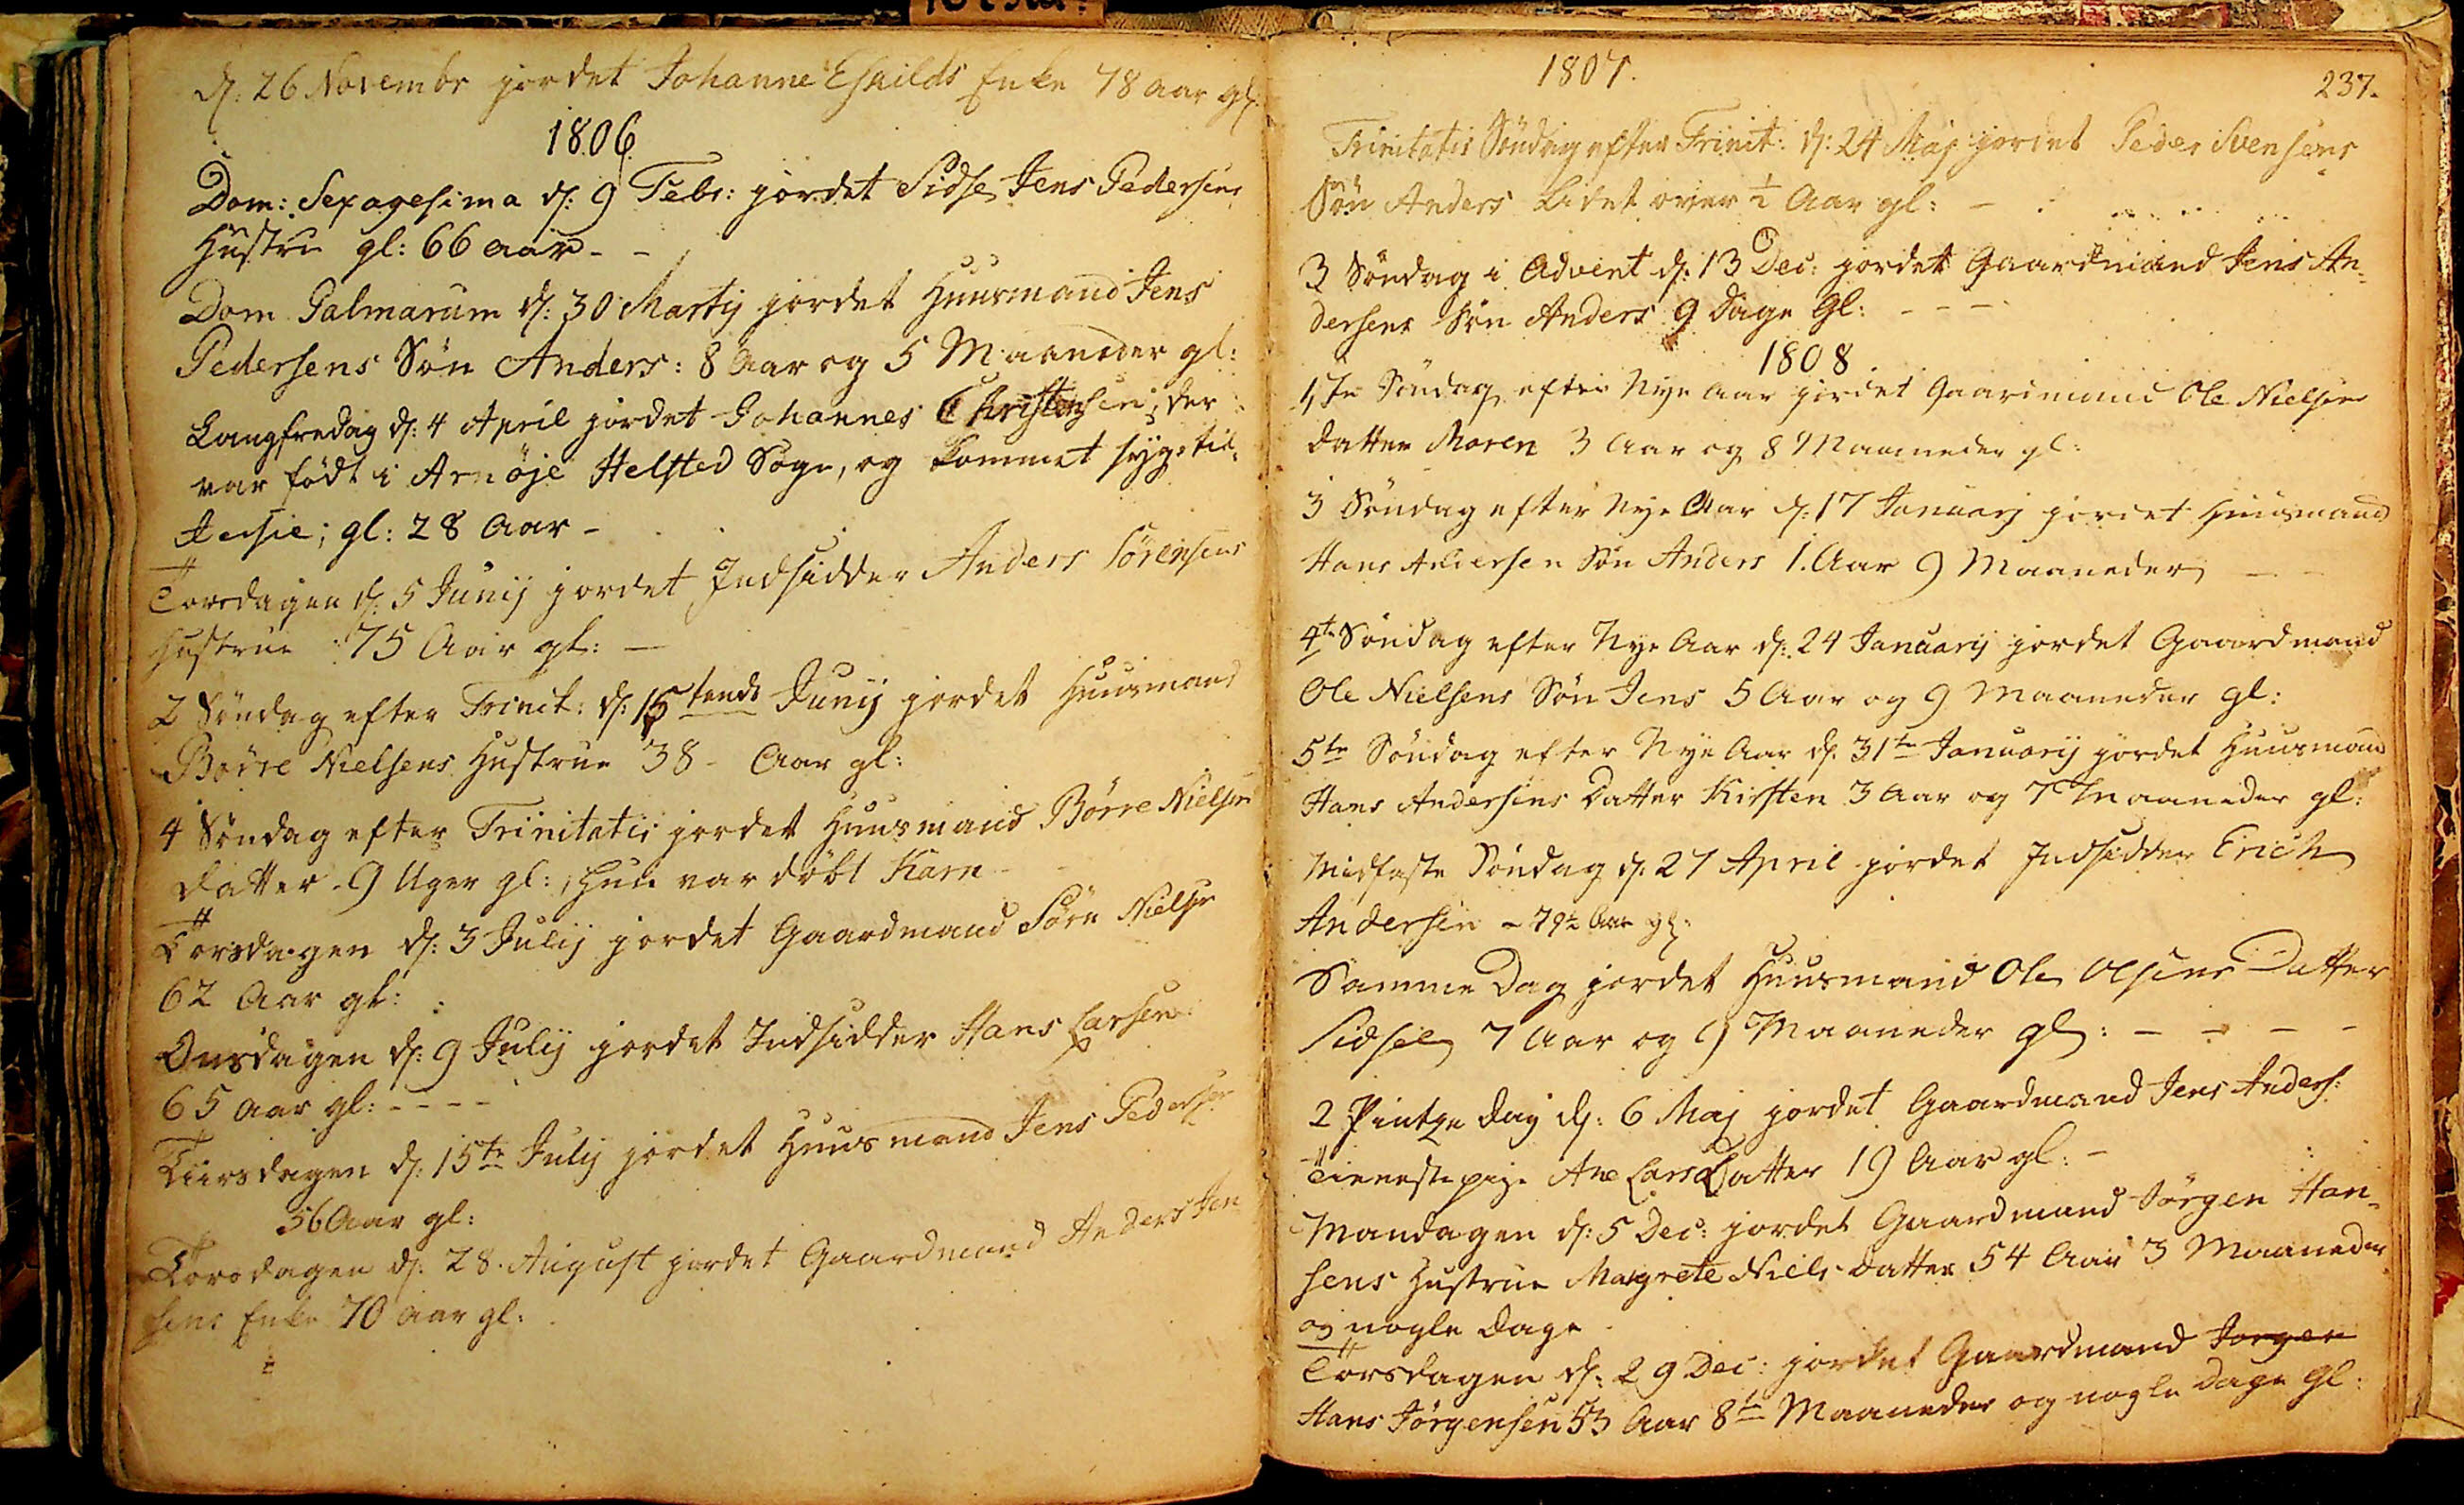d: 26 Novembr jordet Johanne Eskilds Enke 78 Aar gl:
1806
Dom: Sexagesima d: 9 Febr: jordet Sidse Jens Pedersens
Hustru gl: 66 Aar
Dom Palmarum d: 30 Martij jordet Huusmand Jens
Pedersens Søn Anders: 8 Aar og 5 Maaneder gl:
Langfredag d: 4 April jordet Johannes Christensen; der
var født i Arnøje Helsted Sogn, og kommet syg til
Jersie; gl: 28 Aar
Torsdagen d: 5 Junij jordet Indsidder Anders Sørensens
Hustrue 75 Aar gl:
2 Søndag efter Trinit: d: 15tende Junij jordet Huusmand
Børre Nielsens Hustrue 38 Aar gl:
4 Søndag efter Trinitatis jordet Huusmand Børre Nielsens
Datter 9 Uger gl:; hun var døbt Karn
Torsdagen d: 3 Julij jordet Gaardmand Sørn Nielsen
62 Aar gl:
Onsdagen d: 9 Julij jordet Indsidder Hans Larsen
65 Aar gl: 
Tiirsdagen d: 15te Julij jordet Huusmand Jens Pedersen
56 Aar gl:
Torsdagen d: 28 August jordet Gaardmand Anders Jen
sens Enke 70 Aar gl:
237.
1807
Trinitatis Søndag efter Trinit: d: 24 Maj jordet Peder Svensens
Søn Anders Lidet over ½ Aar gl: -
3 Søndag i Advent d: 13 Dec: jordet Gaardmand Jens An¬
dersens Søn Anders 9 Dage gl:
1808.
1ste Søndag efter Nye Aar jordet Gaardmand Ole Nielsens
Datter Maren 3 Aar og 8 Maaneder gl:
3 Søndag efter Nye Aar d: 17 Januarj jordet Huusmand
Hans Andersen Søn Anders 1. Aar 9 Maneder
4te Søndag efter Nye Aar d: 24 Januarij jordet Gaardmand
Ole Nielsens Søn Jens 5 Aar og 9 Maaneder gl:
5te Søndag efter Nye Aar d. 31 Januarij jordet Huusmand
Hans Andersens Datter Kirsten 3 Aar og 7 Maaneder gl:
Midfaste Søndag d: 27 April jordet Indsidder Erich
Andersen - 79½ Aar gl:
Samme Dag jordet Huusmand Ole Olsens Datter
Sidsel 7 Aar og 9 Maaneder gl:
2 Pintze Dag d: 6 Maj jordet Gaardmand Jens Anders:
Tienestepige Ane Lars Datter 19 Aar gl:
Mandagen d: 5 Dec: jordet Gaardmand Jørgen Han¬
sens Hustrue Margrete Niels Datter 54 Aar 3 Maaneder 
og nogle Dage.
Torsdagen d: 29 Dec: jordet Gaardmand Jørgen
Hans Jørgensen 53 Aar 8te Maaneder og nogle Dage gl:
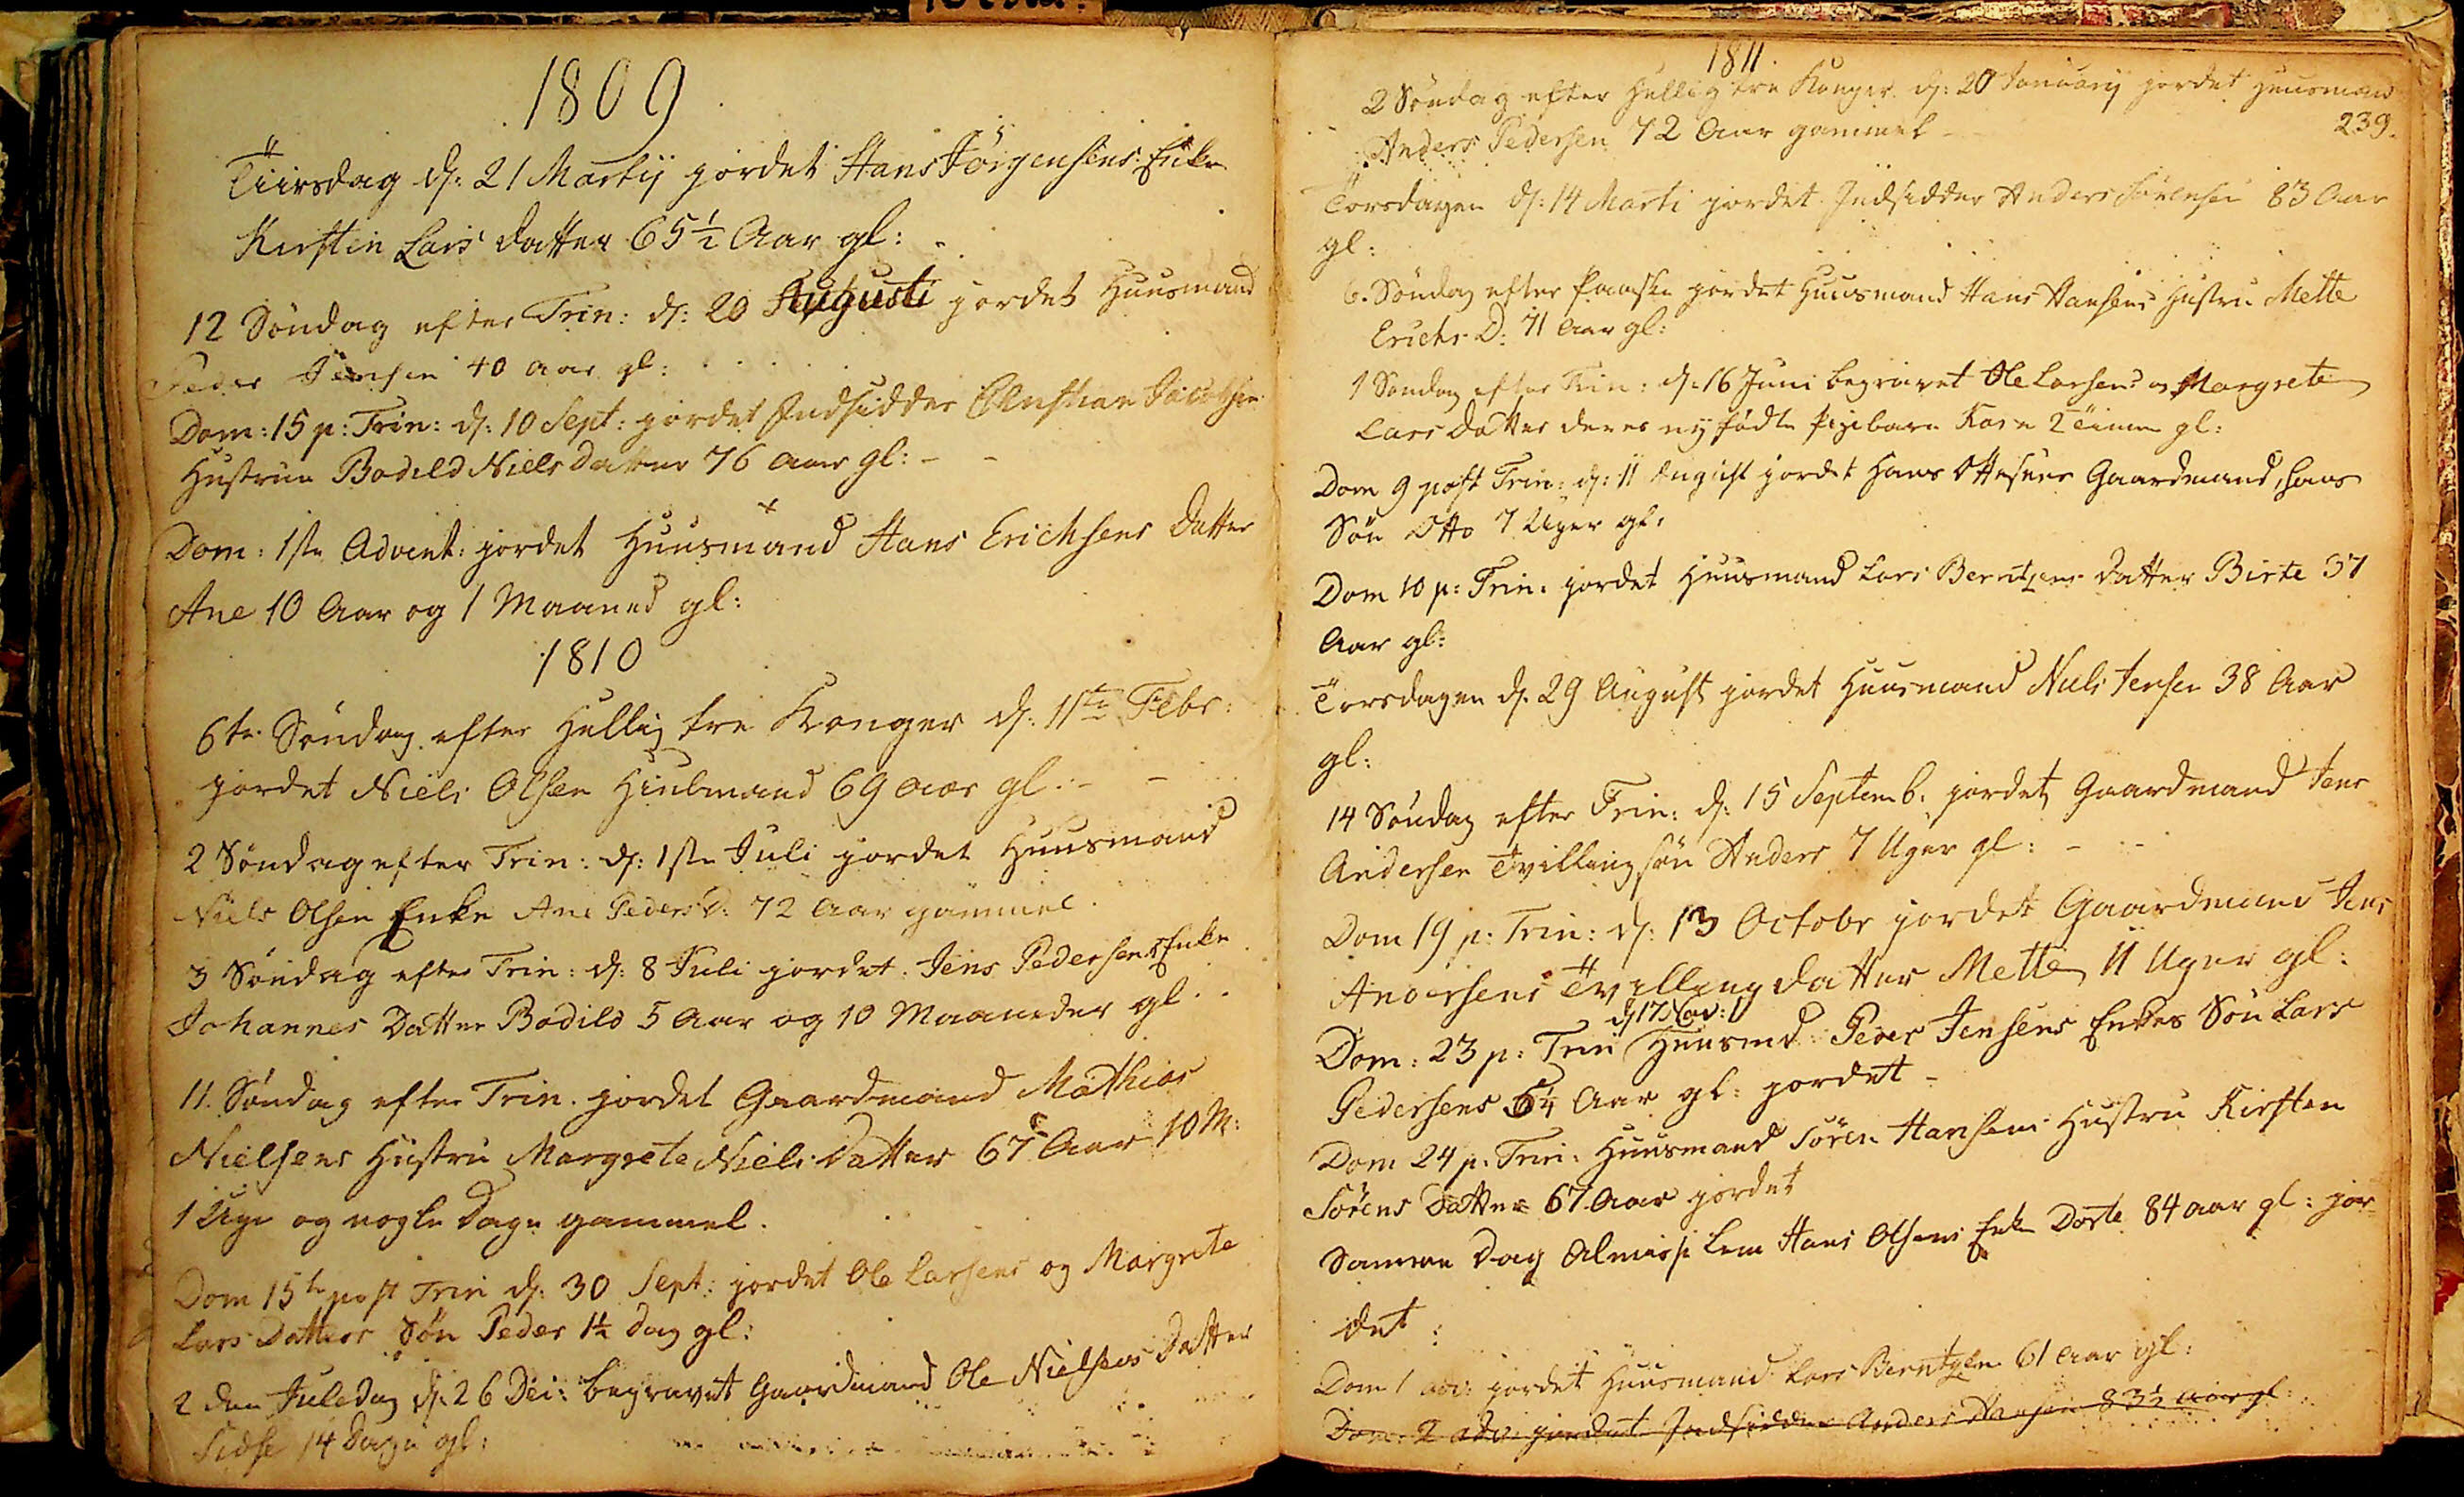1809
Tirsdagen d: 21 Martij jordet Hans Jørgensens Enke
Kirsten Lars Datter 65½ Aar gl:
12 Søndag efter Trin: d: 20 Augusti jordet Huusmand
Peder Jensen 40 Aar gl:
Dom: 15 p: Trin: d: 10 Sept. jordet Indsidder Christian Jacobsen
Hustrue Bodild Niels Datter 76 Aar gl:
Dom: 1ste Advent: jordet Huusmand Hans Erichsens Datter
Ane 10 Aar og 1 Maaned gl:
1810
6te. Søndag efter Hellig tre Konger d. 11te Febr:
jordet Niels Olsen Hiulmand 69 Aar gl.
2 Søndag efter Trin: d. 1ste Juli jordet Huusmand
Niels Olsens Enke Ane Peders D: 72 Aar gammel.
3 Søndag efter Trin: d: 8 Juli jordet: Jens Pedersens Enke
Johannes Datter Bodild 5 Aar og 10 Maaneder gl.
11. Søndag efter Trin. jordet Gaardmand Mathias
Nielsens Hustru. Margrete Niels Datter 67 Aar 10 M:
1 Uge og nogle Dage gammel.
Dom 15te post Trin d: 30 Sept: jordet Ole Larsens og Margrete
Lars Datters Søn Peder 1½ Dag gl:
2den Juledag d: 26 Dec: begravet Gaardmand Ole Nielsens Datter
Sidse 14 Dage gl:
239.
1811
2 Søndag efter Hellig tre Konger d. 20 Januarij jordet Huusmand
Anders Pedersen 72 Aar gammel
Torsdagen d: 14 Marti jordet Indsidder Anders Sørensen 83 Aar
gl:
6. Søndag efter Paaske jordet Huusmand Hans Hansens Hustru Mette
Erichs D: 71 Aar gl:
1. Søndag efter Trin: d: 16 Juni begravet Ole Larsens og Margrete
Lars Datter deres nyfødte Pigebarn Karn 2 Timer gl:
Dom 9 post Trin: d: 11 August jordet Hans Ottesens Gaardmand, hans
Søn Otto 7 Uger gl:
Dom 10 p: Trin: jordet Huusmand Lars Berntzens Datter Birte 37
Aar gl:
Torsdagen d. 29 August jordet Huusmand Niels Jensen 38 Aar
gl:
14 Søndag efter Trin: d: 15 Septemb: jordet Gaardmand Jens
Andersens Tvillingsøn Anders 7 Uger gl:
Dom 19 p: Trin: d: 13 Octobr jordet Gaardmand Jens
Andersens Tvillingdatter Mette 11 Uger gl:
d 17 Nov:
Dom: 23 p: Trin Huusmd: Peder Jensens Enkes Søn Lars
Pedersens 5 1/4 Aar gl: jordet
Dom 24 p: Trin: Huusmand Søren Hansens Hustru Kirsten
Sørens Datter 67 Aar jordet
Samme dag Almisse Lem Hans Olsens Enke Dorte 84 Aar gl: jor¬
det.
Dom 1 Adv: jordet Huusmand Lars Berntzen 61 Aar gl:
Dom: 2 Adv: jordet Indsidder Anders Hansen 83½ Aar gl: